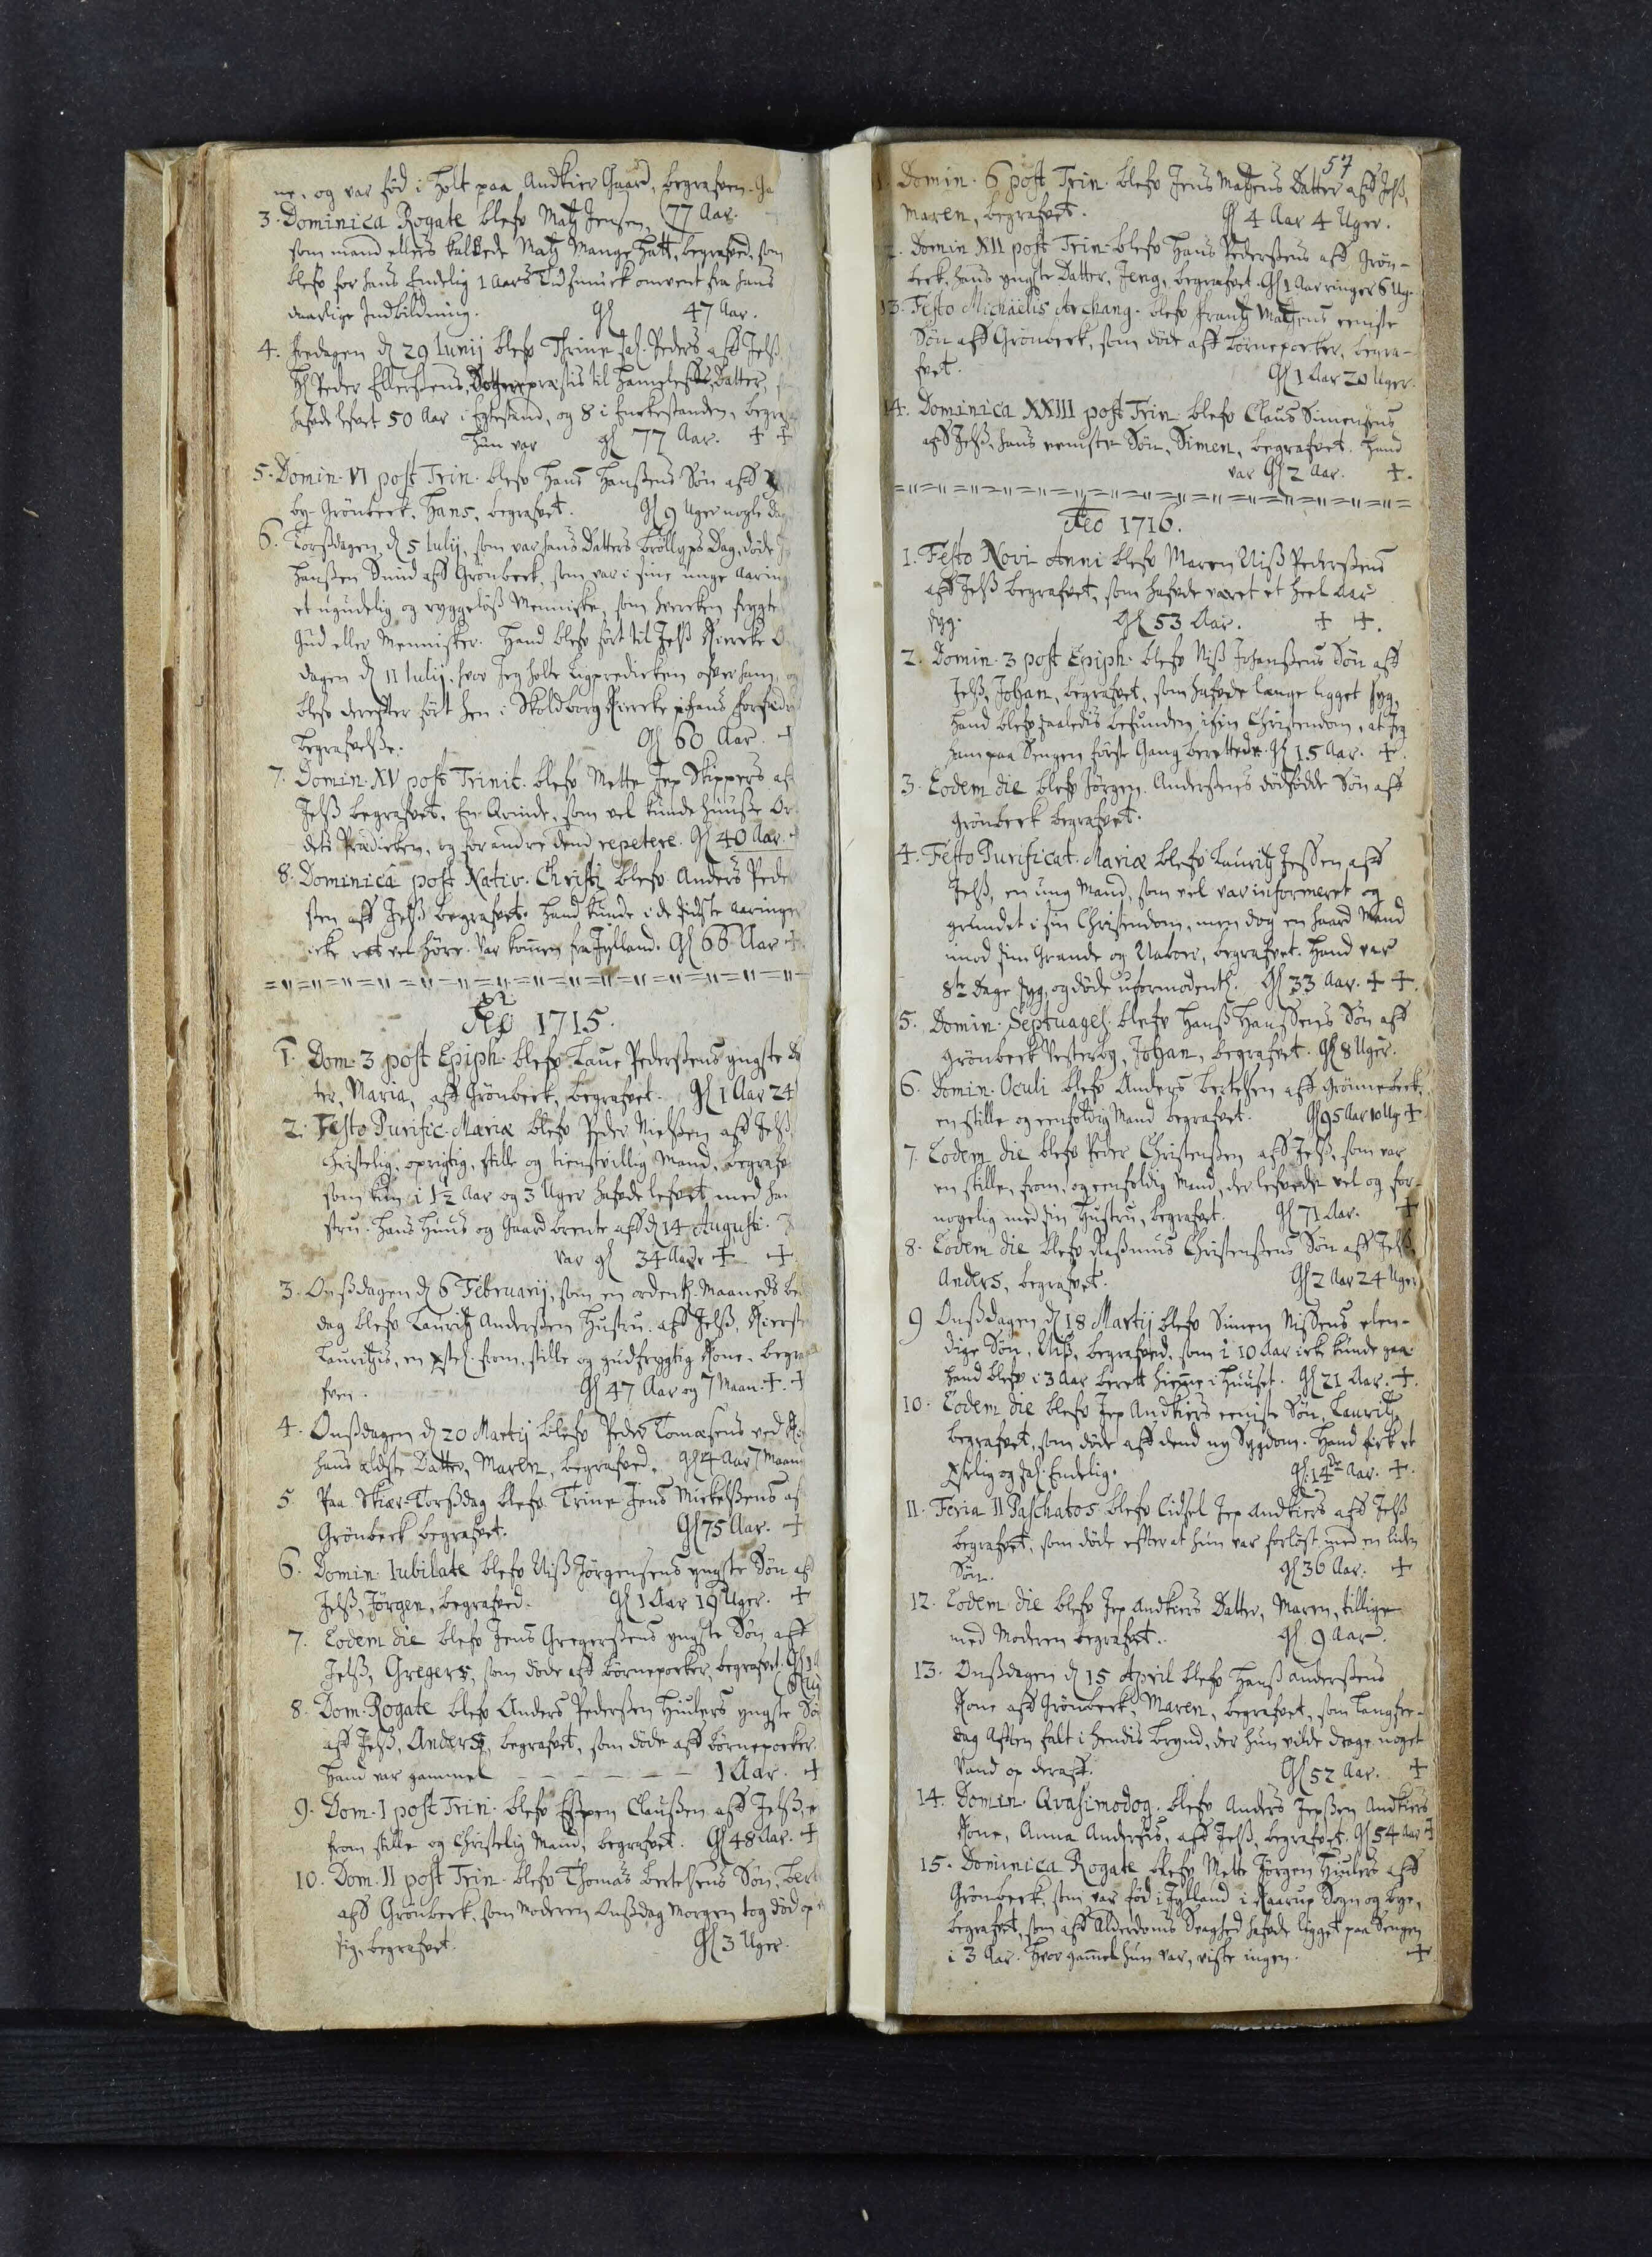ne, og var fød i Holt paa Andkier Gaard, begrafven
77 Aar.
3. Dominica Rogate blefv Matz Jensen,
som mand ellers kaldede Matz Mange Hatt, begrafved, som
blefv for hans Endelig 1 Aars tid smuck omværet fra hans
daarlige Indbildning.
Gl 47 Aar.
4. Fredagen d 29 Iunij blefv Thrine sal. Peders aff Jelss
Hl Peder Ellersens, Sogneprastis til Hameleffs Datter,
hafvde lefvet 50 Aar i Egtestand, og 8 i Enckestanden, begraf
Hun var gl 77 Aar.
5. Domin. VI post Trin. blefv Hans Hanssens Søn aff
by- Grønbeck, Hans begrafvet. Gl 9 Uger nogle Dag
6. Torssdagen d 5 Iulij, som var hans Datters Brøllyps Dag døde
Hansen Smid aff Grønbeck, som var i sine unge Aaring 
et ugudelig og ryggeløss Menniske, som hvercken frygte 
Gud eller Mennisker. Hand blefv ført til Jelss Kiercke
dagen d 11 Iulij, hvor Jeg holte Ligpredicken ofver ham og
blefv dereffter ført hen i Skoldborg Kiercke j hans forfadr 
Begrafvelsse.
Gl 60 Aar.
7. Domin. XV post Trinit. blefv Mete Jep Skippers af
Jelss begrafvet. En Qvinde, som vel kunde huuske Or¬
dets Prædicken, og for andre dend repetere. Gl 40 Aar.
8. Dominica post Nativ. Christi blefv Anders Pede
ssen aff Jelss begrafvet. Hand kunde i de sidste Aaringer
icke ret vel høre. Var kommen fra Jylland. Gl 66 Aar
Ao 1715
1. Dom. 3 post Epiph. blefv Laue Pederssens yngste Da 
ter, Maria, aff Grønbeck, begrafvet. Gl 1 Aar 24
2. Festo Purific. Maria blefv Peder Nielssens aff Jels
christelig, oprigtig, stille og tienstvillig Mand, begrafv
som kun i 1½ Aar og 3 Uger hafvde lefvet med ha
stru. Hans Huus og Gaard brente aff d 14 Augusti.
var gl 34 Aar
3. Onssdagen d 6 Februarij, som er orden## Maaneds be 
dag blefv Lauritz Anderssen Hustru aff Jelss, Kierste 
Lauritzis, en xstel. from, stille og gudfrygtig Kone, begr 
fven.
Gl 47 Aar og 7 Maan.
4. Onssdagen d 20 Martij blefv Peder Thomasens ved
hans aldste Datter, Maren, begrafved. Gl 4 Aar 7 Maane 
5. Paa Skiær-Torssdag blefv Trine Jens Mickelssens af
Grønbeck begrafvet.
Gl 75 Aar.
6. Domin. Iubilate blefv Niss Jørgensens yngste Søn aff
Jelss, Jørgen, begrafved. Gl 1 Aar 19 Uger.
7. Eodem die blefv Jens Gregerssens yngste Søn aff
Jelss, Gregers, som døde aff børnepocker, begrafvet. Gl 1
8. Dom. Rogate blefv Anders Pederssen Hiulers yngste Sø 
aff Jelss, Anders, begrafvet, som døde aff børnepocker.
Hand var gammel
1 Aar.
9. Dom. I post Trin. blefv Esspen Claussen aff Jelss,
from stille og christelig Mand, begrafvet. Gl 48 Aar.
10. Dom. II post Trin. blefv Thomas Bertelsens Søn, Ber
aff Grønbeck, som Moderen Onssdag Morgen tog op
sig, begrafvet.
Gl 3 Uger.
57
 1. Domin. 6 post Trin. blefv Jens Matzens Datter aff Jelss,
Maren, begrafvet.
Gl 4 Aar 4 Uger.
2. Domin. XII post Trin. blefv Hans Pederssens aff Grøn¬
beck, hans yngste Datter, Jeng, begrafvet. Gl 1 Aar ringer 6 Ug
13. Festo Michaelis Archang. blefv Frantz Matzens eeniste
Søn aff Grønbeck, som døde aff børnepocker, begra¬
fvet.
Gl 1 Aar 20 Uger.
14. Dominica XXIII post Trin. blefv Claus Simensens
aff Jels, hans eeniste Søn, Simen, begrafvet. Hand
var Gl 2 Aar.
Ao 1716.
1. Festo Novi Anni blefv Maren Niss Pederssens
aff Jelss begrafvet, som hafvde varet et heel Aar
syg.
Gl 53 Aar.
2. Domin. 3 post Epiph. blefv Niss Johanssens Søn aff
Jelss, Johan, begrafvet, som hafvde længe ligget syg,
hand blefv saaledis befunden isin Christendom, at Jeg
ham paa Sengen første Gang berettede. Gl 15 Aar.
3. Eodem die blefv Jørgen Anderssens dødfødde Søn aff
Grønbeck begrafvet.
4. Festo Purificat. Maria blefv Lauritz Jessen aff
Jelss, en ung Mand, som vel var informeret og
grundet i sin Christendom, men dog en haard Mand
imod sine Grande og Naboer, begrafet. Hand var
8te Dage syg, og døde uformodentl. Gl 33 Aar.
5. Domin. Septuages. blefv Hanss Hanssens Søn aff
Grønbeck Vesterby, Johan, begrafvet. Gl 8 Uger.
6. Domin. Oculi blefv Anders Bertelsen aff Grønnebeck,
en stille og eenfoldig Mand begrafet. Gl 95 Aar 10 Ug
7. Eodem die blefv Peder Christenssen aff Jelss, som var
en stille, from, og eenfoldig Mand, der lefvede vel og for¬
noyelig med sin hustru, begrafvet. Gl 71 Aar.
8. Eodem die blefv Rassmus Christenssens Søn aff Jelss,
Anders, begrafvet.
Gl 2 Aar 24 Uger
9. Onssdagen d 18 Martij blefv Simen Nissens elen¬
dige Søn, Niss, begrafved, som i 10 Aar icke kunde gaa.
hand blefv i 3 Aar beret hiemme i huuset. Gl 21 Aar.
10. Eodem die blefv Jep Andkiers eeniste Søn, Lauritz,
begrafvet, som døde aff dend ny Sygdom. Hand fick et
xtelig og sal. Endelig.
Gl 14de Aar
11. Feria II Paschatos blefv Cidsel Jep Andkiers aff Jelss
begrafvet, som døde effter at hun var forløst med en liden
søn.
Gl 36 Aar.
12. Eodem die blefv Jep Andkiers Datter, Maren, tillige
med Moderen begrafvet.
Gl 9 Aar
13. Onssdagen d 15 April blefv Hanss Anderssens
Kone aff Grønbeck, Maren, begrafvet, som Langfre¬
dag Aften falt i hendis brynd, der hun vilde drage noget
Vand op deraff.
Gl 52 Aar.
14. Domin. Qvasimodog. blefv Anders Jepssen Andkiers
Kone, Anna Andersis, aff Jelss, begrafvet. Gl 54 Aar
15. Dominica Rogate blefv Mette Jørgen Hiulers aff
Grønbeck, som var fød i Jylland i Kaarup Sogn og bye,
begrafvet, som aff Alderdoms Svaghed hafvde ligget paa Sengen
i 3 Aar. Hvor gamel hun var, viste ingen.